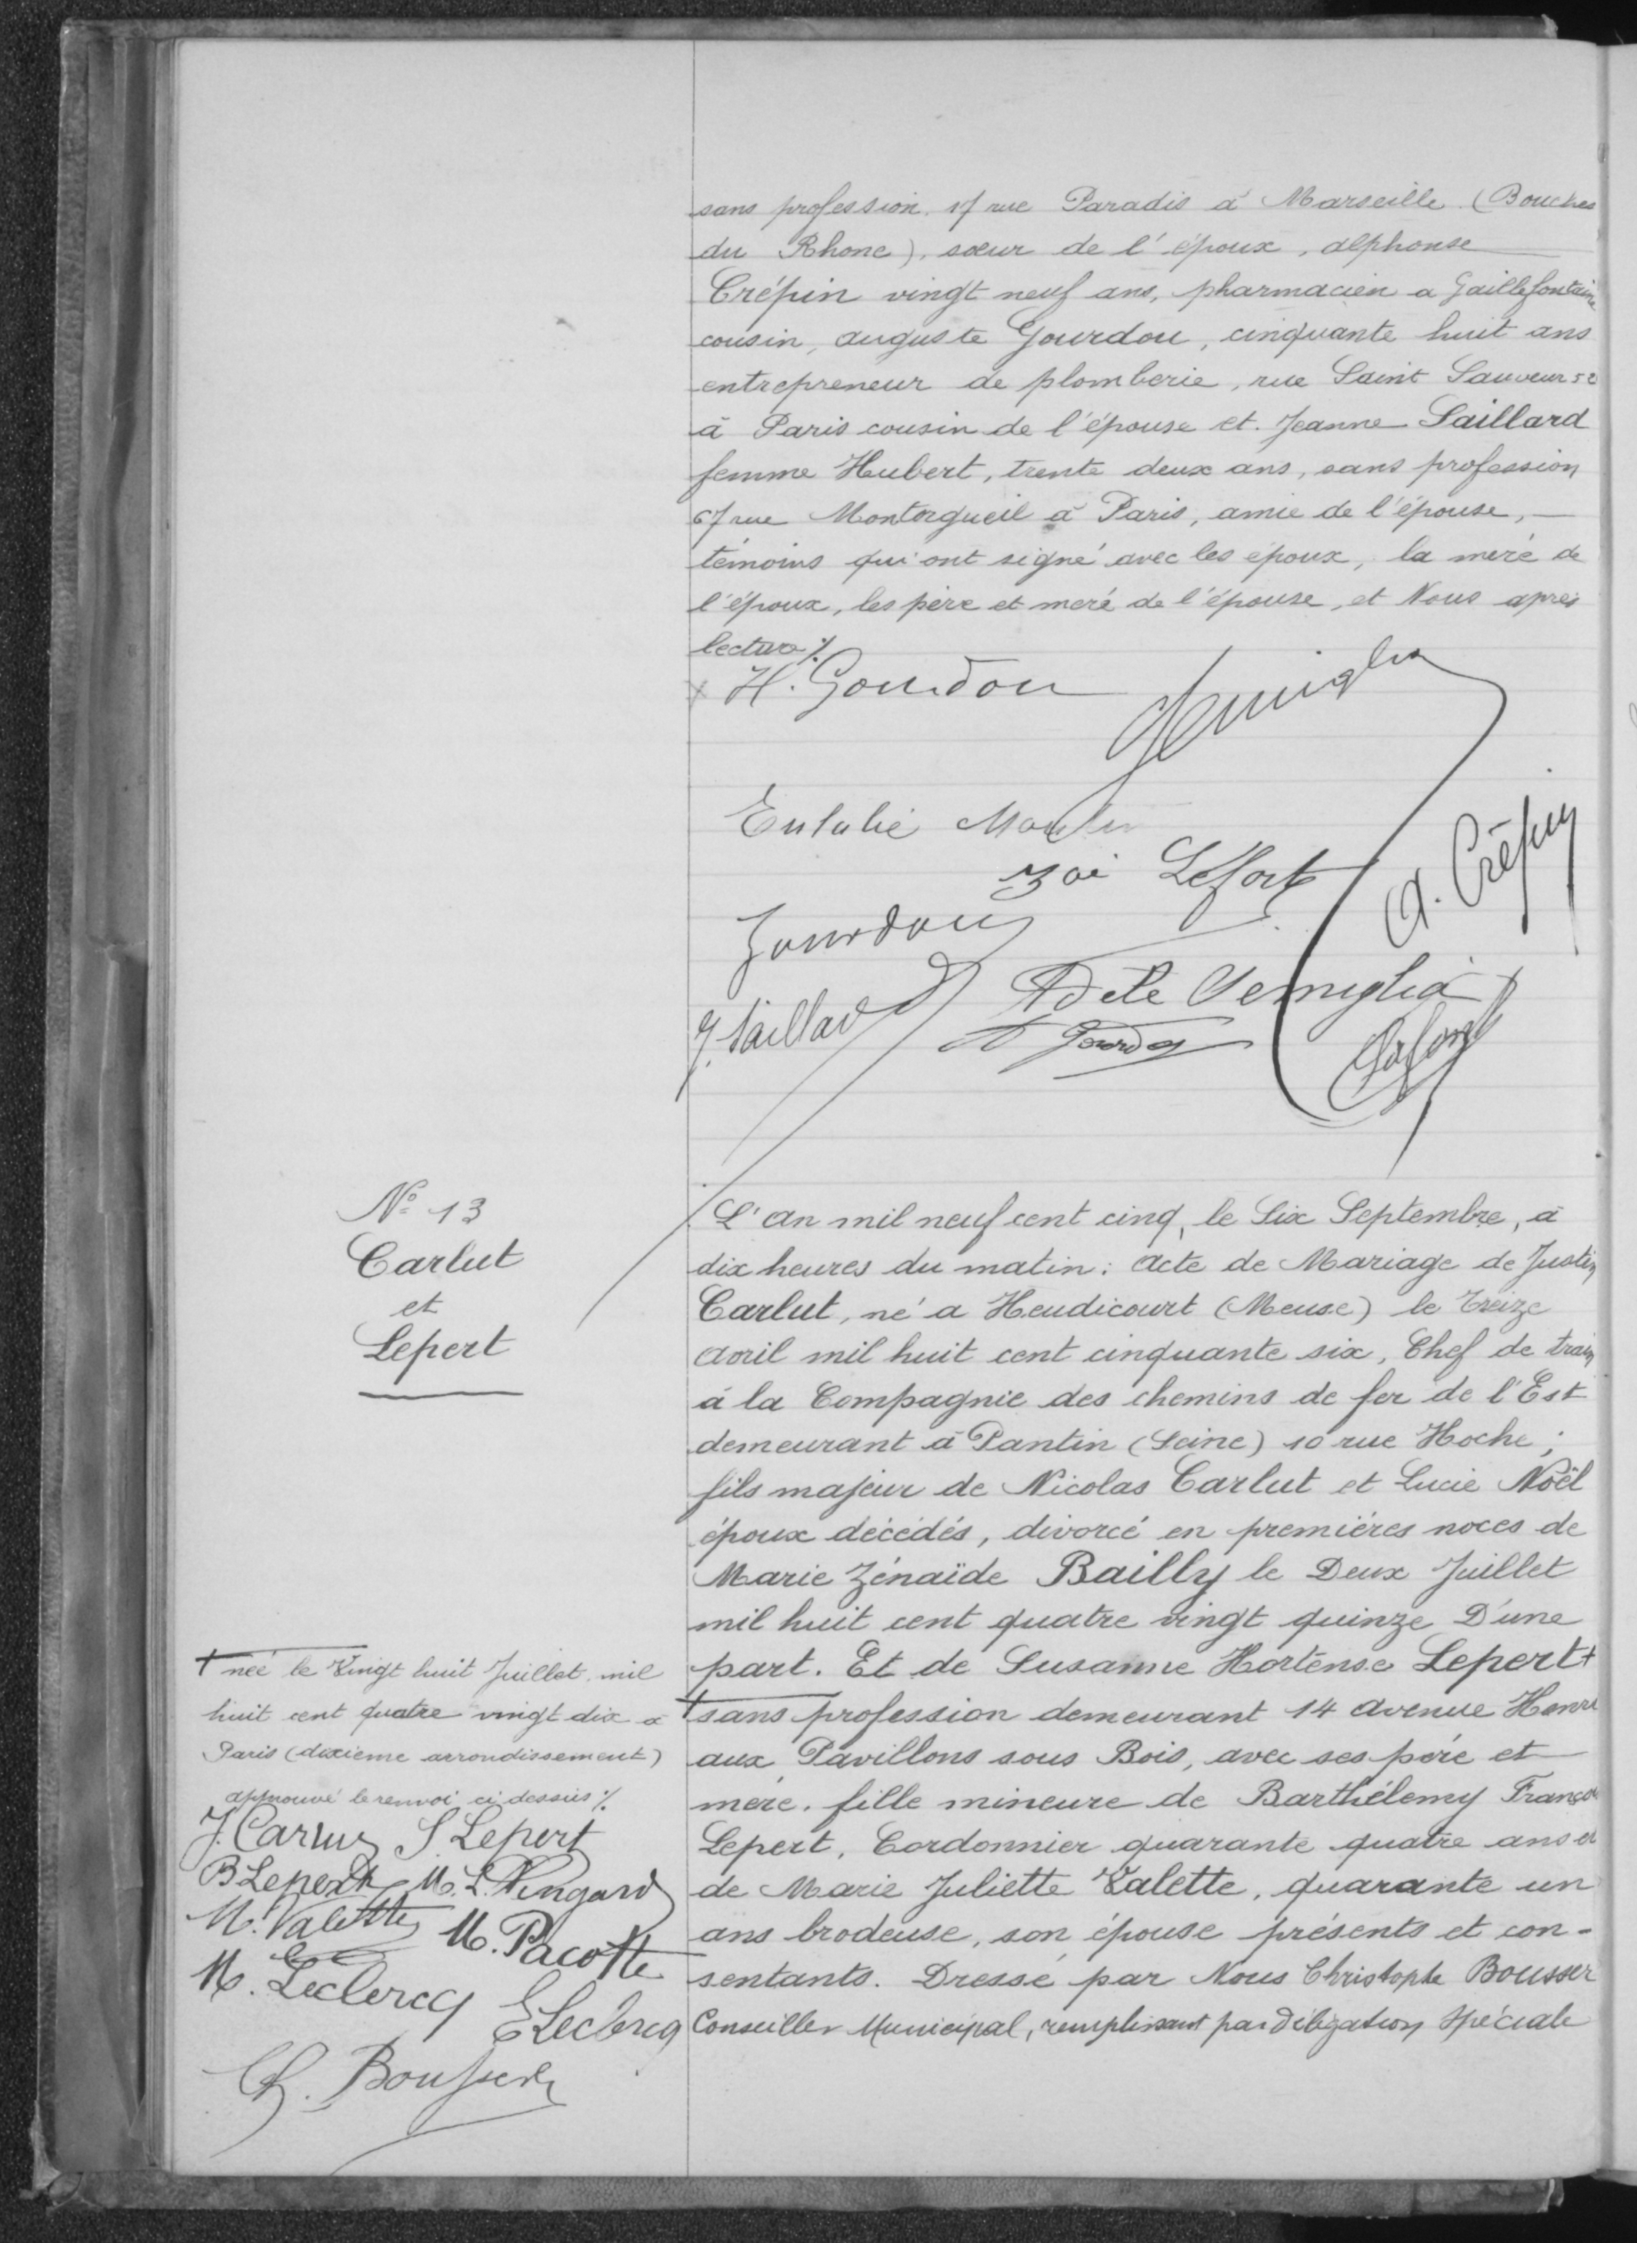sans profession, 17 rue Paradis à Marseille (Bouches
du Rhone), soeur de l'époux, Alphonse
Crépin vingt neuf ans, pharmacien à Gaillefontaine
cousin, auguste Gourdou, cinquante huit ans
entrepreneur de plomberie, rue Saint Sauveur né
à Paris cousin de l'épouse et Jeanne Saillard
femme Hubert, trente deux ans, sans profession
67 rue Montorgueil à Paris, amie de l'épouse,
témoins qui ont signé avec les époux, la mère de
l'époux, les père et mère de l'épouse et Nous apres
lecture ./.
N°13
Carlut
et
Lepert
L'An mil neuf cent cinq, le Six Septembre, à
dix heures du matin: Acte de Mariage de Justin
Carlut, né à Heudicourt (Meuse) le Treize
Avril mil huit cent cinquante six, Chef de train
à la Compagnie des chemins de fer de l'Est
demeurant à Pantin (Seine) 10 rue Hoche;
fils majeur de Nicolas Carlut et Lucie Noël
époux décédés, divorcé en premières noces de
Marie Zénaïde Bailly le Deux Juillet
mil huit cent quatre vingt quinze D'une
part. Et de Susanne Hortense Lepert
sans profession demeurant 14 Avenue Henri
aux Pavillons sous Bois, avec ses père et
mère, fille mineure de Barthélemy François
Lepert, Cordonnier quarante quatre ans et
de Marie Juliette Valette, quarante un
ans brodeuse, son épouse présents et con-
sentants. Dressé par Nous Christophe Bousser
Conseiller Municipal, remplissant par délégation spéciale
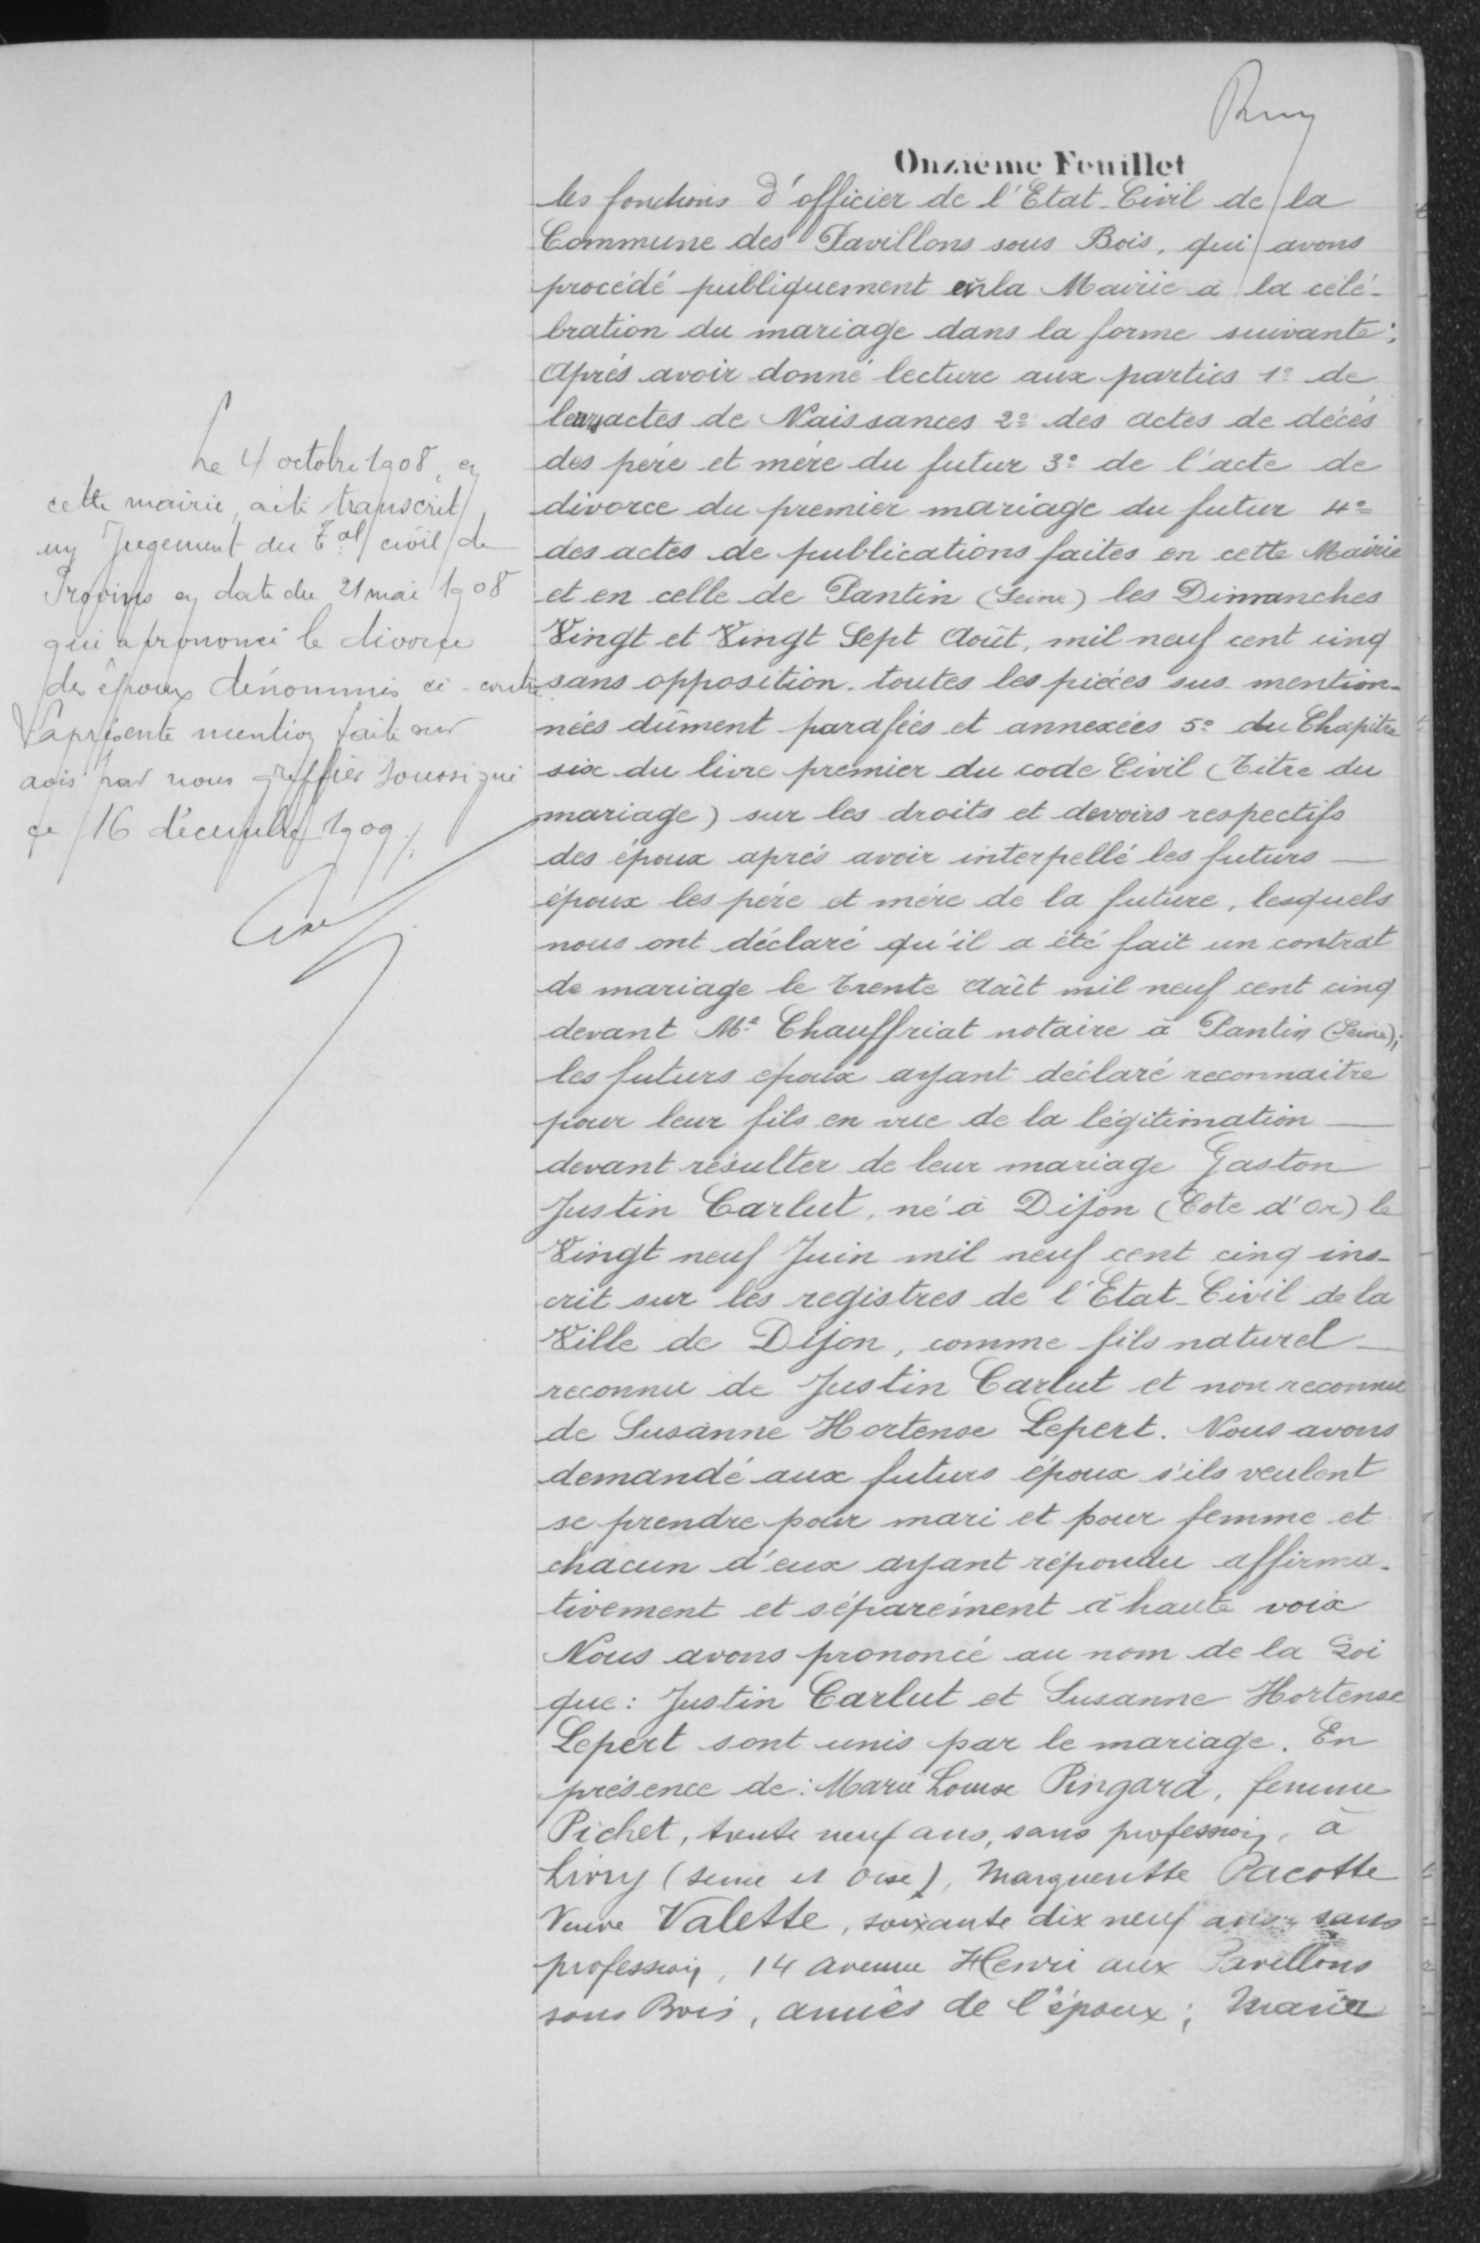les fonctions d'officier de l'Etat Civil de la
Commune des Pavillons sous Bois, qui avons
procédé publiquement en la Mairie à la célé-
bration du mariage dans la forme suivante:
Après avoir donné lecture aux parties 1° de
leurs actes de Naissances 2° des actes de décès
des père et mère du futur 3° de l'acte de
divorce du premier mariage du futur 4°
des actes de publications faites en cette Mairie
et en celle de Pantin (Seine) les Dimanches
Vingt et Vingt Sept Août mil neuf cent cinq
sans opposition, toutes les pièces sus mention-
nées dûment parafées et annexées 5° du Chapitre
six du livre premier du code Civil (Titre du
mariage) sur les droits et devoirs respectifs
des époux après avoir interpellé les futurs
époux les père et mère de la future, lesquels
nous ont déclaré qu'il a été fait un contrat
de mariage le Trente Août mil neuf cent cinq
devant Me Chauffriat notaire à Pantin (Seine);
les futurs epoux ayant déclaré reconnaître
pour leur fils en vue de la légitimation
devant résulter de leur mariage Gaston
Justin Carlut, né à Dijon (Cote d'Or) le
Vingt neuf Juin mil neuf cent cinq ins-
crit sur les registres de l'Etat Civil de la
Ville de Dijon, comme fils naturel
reconnu de Justin Carlut et non reconnu
de Susanne Hortense Lepert. Nous avons
demandé aux futurs époux s'ils veulent
se prendre pour mari et pour femme et
chacun d'eux ayant répondu affirma-
tivement et séparément à haute voix
Nous avons prononcé au nom de la loi
que: Justin Carlut et Susanne Hortense
Lepert sont unis par le mariage. En
présence de: Marie Louise Pingard, femme
Pichet, trente neuf ans, sans profession, à
Livry (Seine et Oise), Marguerite Pacotte
Veuve Valette, soixante dix neuf ans sans
profession, 14 avenue Henri aux Pavillons
sous Bois, amies de l'époux; Marie